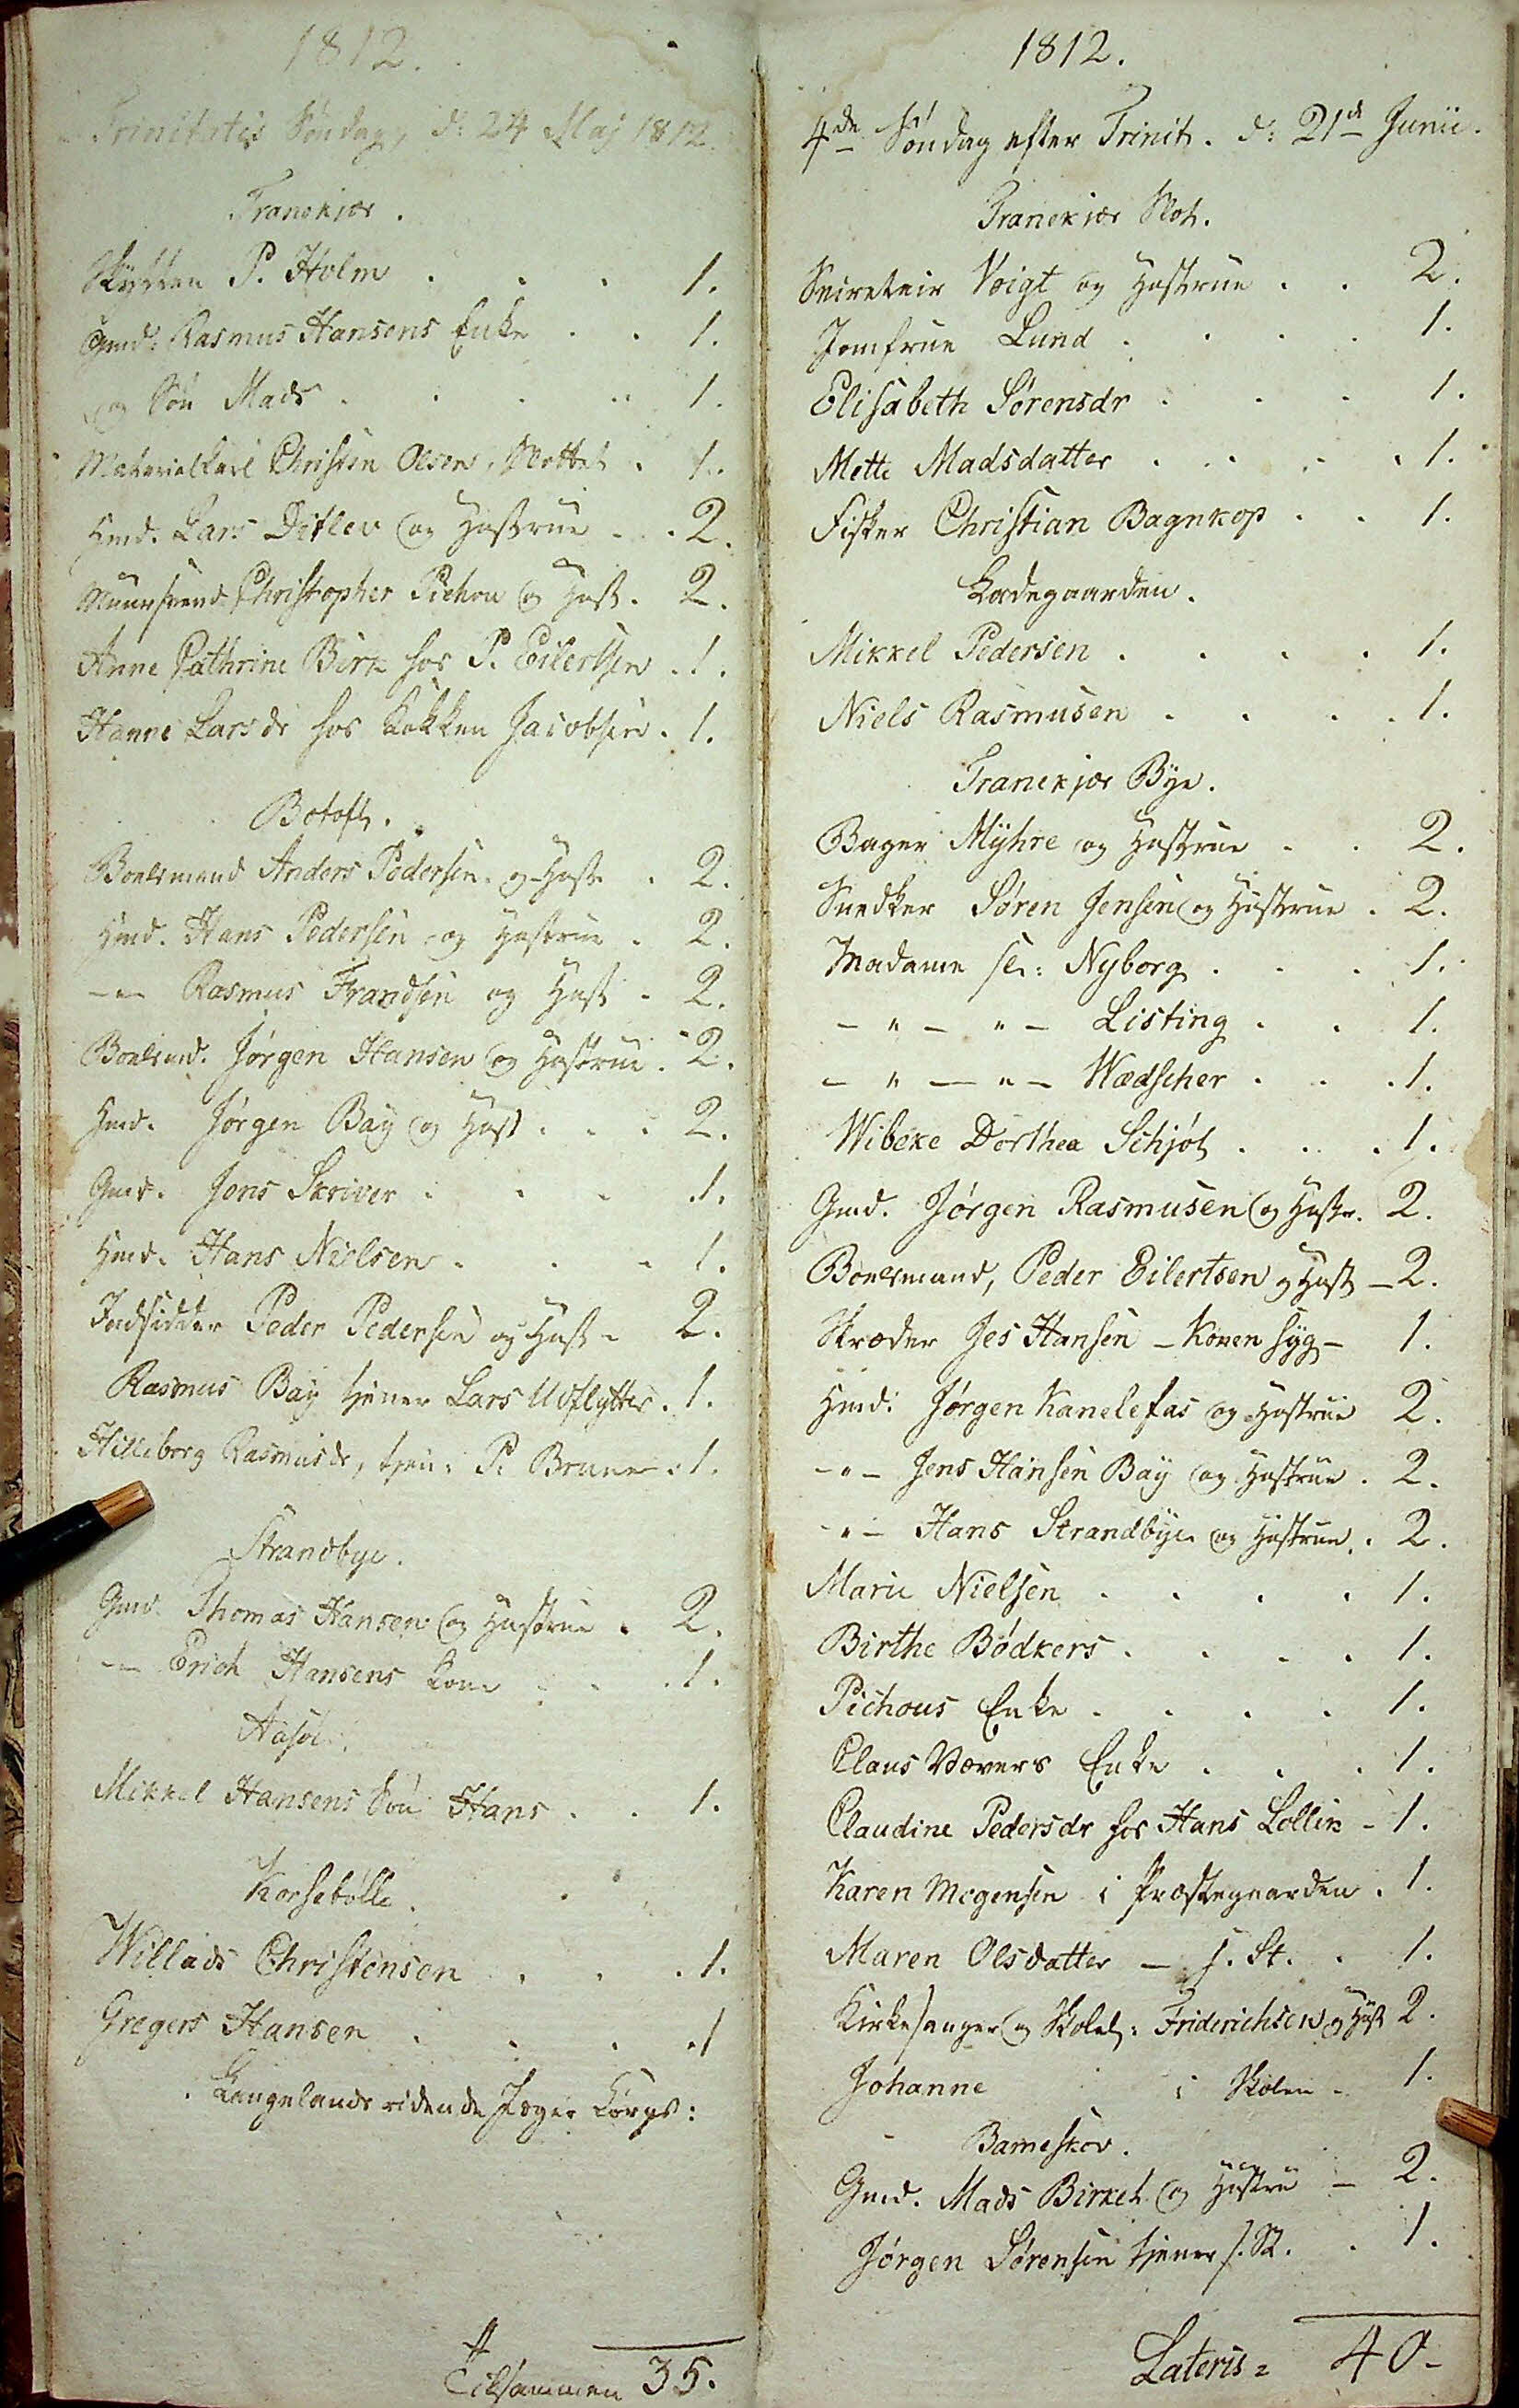1812.
Trinitatis Søndag d: 24 Maj 1812
Tranekjær
Skytten P. Holm . . . 1.
Gmd: Rasmus Hansens Enke . . 1.
og Søn Mads . . . . . 1.
Materialkarl Christen Olsen, Slottet . 1.
Hmd. Lars Ditlev og Hustrue . . . 2
Muursvend Christopher Pichou og Hust . 2.
Anne Cathrine Børk hos P. Eilertsen . 1.
Hanne Larsdr hos Kokken Jacobsen . 1.
Botoft.
Boelsmand Anders Pedersen og Hustr . 2
Hmd. Hans Pedersen og Hustrue . 2
-''- Rasmus Frandsen og Hust . 2.
Boelsmd. Jørgen Hansen og Hustrue . 2
Hmd. Jørgen Bay og Hust . . . 2.
Gmd. Jens Skriver . . . . 1.
Hmd. Hans Nielsen . . . 1.
Indsidder Peder Pedersen og Hust . 2.
Rasmus Bay tjener Lars Udflytter . 1.
Hilleborg Rasmusdr, tjen: P: Brunm . 1.
Strandbye.
Gmd. Thomas Hansen og Hustrue . 2.
-"- Erich Hansens Kone . . 1.
Aasøe
Mikkel Hansens Søn Hans . . 1.
Korsebølle
Willads Christensen . . . 1.
Gregers Hansen . . . . 1
Langelands  ridende Jæger Corps:
Tilsammen 35.
1812.
4de Søndag efter Trinit. d: 21de Junii
Tranekjær Slot.
Secreteir Voigt og Hustrue . . 2.
Jomfrue Lana . . . . 1.
Elisabeth Sørensdr . . . 1.
Mette Madsdatter . . . . . . 1.
Fisker Christian Bagnkop . . 1.
Ladegaarden.
Mikkel Pedersen . . . . 1.
Niels Rasmusen . . . . 1.
Tranekjær Bye.
Bager Myhre, og Hustrue . . 2.
Snedker Søren Jensen og Hustrue . 2.
Madame Sl: Nyborg . . . 1.
-"-"- Listing . . 1.
-"-"- Wædscher . . . 1.
Wibeke Dorthea Schjøt . . . 1.
Gmd. Jørgen Rasmusen og Hustr . 2.
Boelsmand, Peder Eilertsen og Hust - 2.
Skræder Jes Hansen - Konen syg - 1.
Hmd: Jørgen Kanelefas og Hustrue 2.
-"- Jens Hansen Bay og Hustrue . 2.
-"- Hans Strandbye og Hustrue . 2.
Marie Nielsen . . . . 1.
Birthe Bødkers . . . . 1.
Pichous Enke . . . . 1.
Claus Vævers Enke . . . 1.
Claudine Pedersdr hos Hans Lollik - 1.
Karen Mogensen i Præstegaarden . 1.
Maren Olsdatter - s. St . . 1.
Kirkesanger og Skolel: Friderichsen og Hust 2.
Johanne i Skolen - 1.
Bameskov
Gmd. Mads Birket og Hustru - 2.
Jørgen Sørensen tjener  s##. St . . 1.
Lateris = 40.
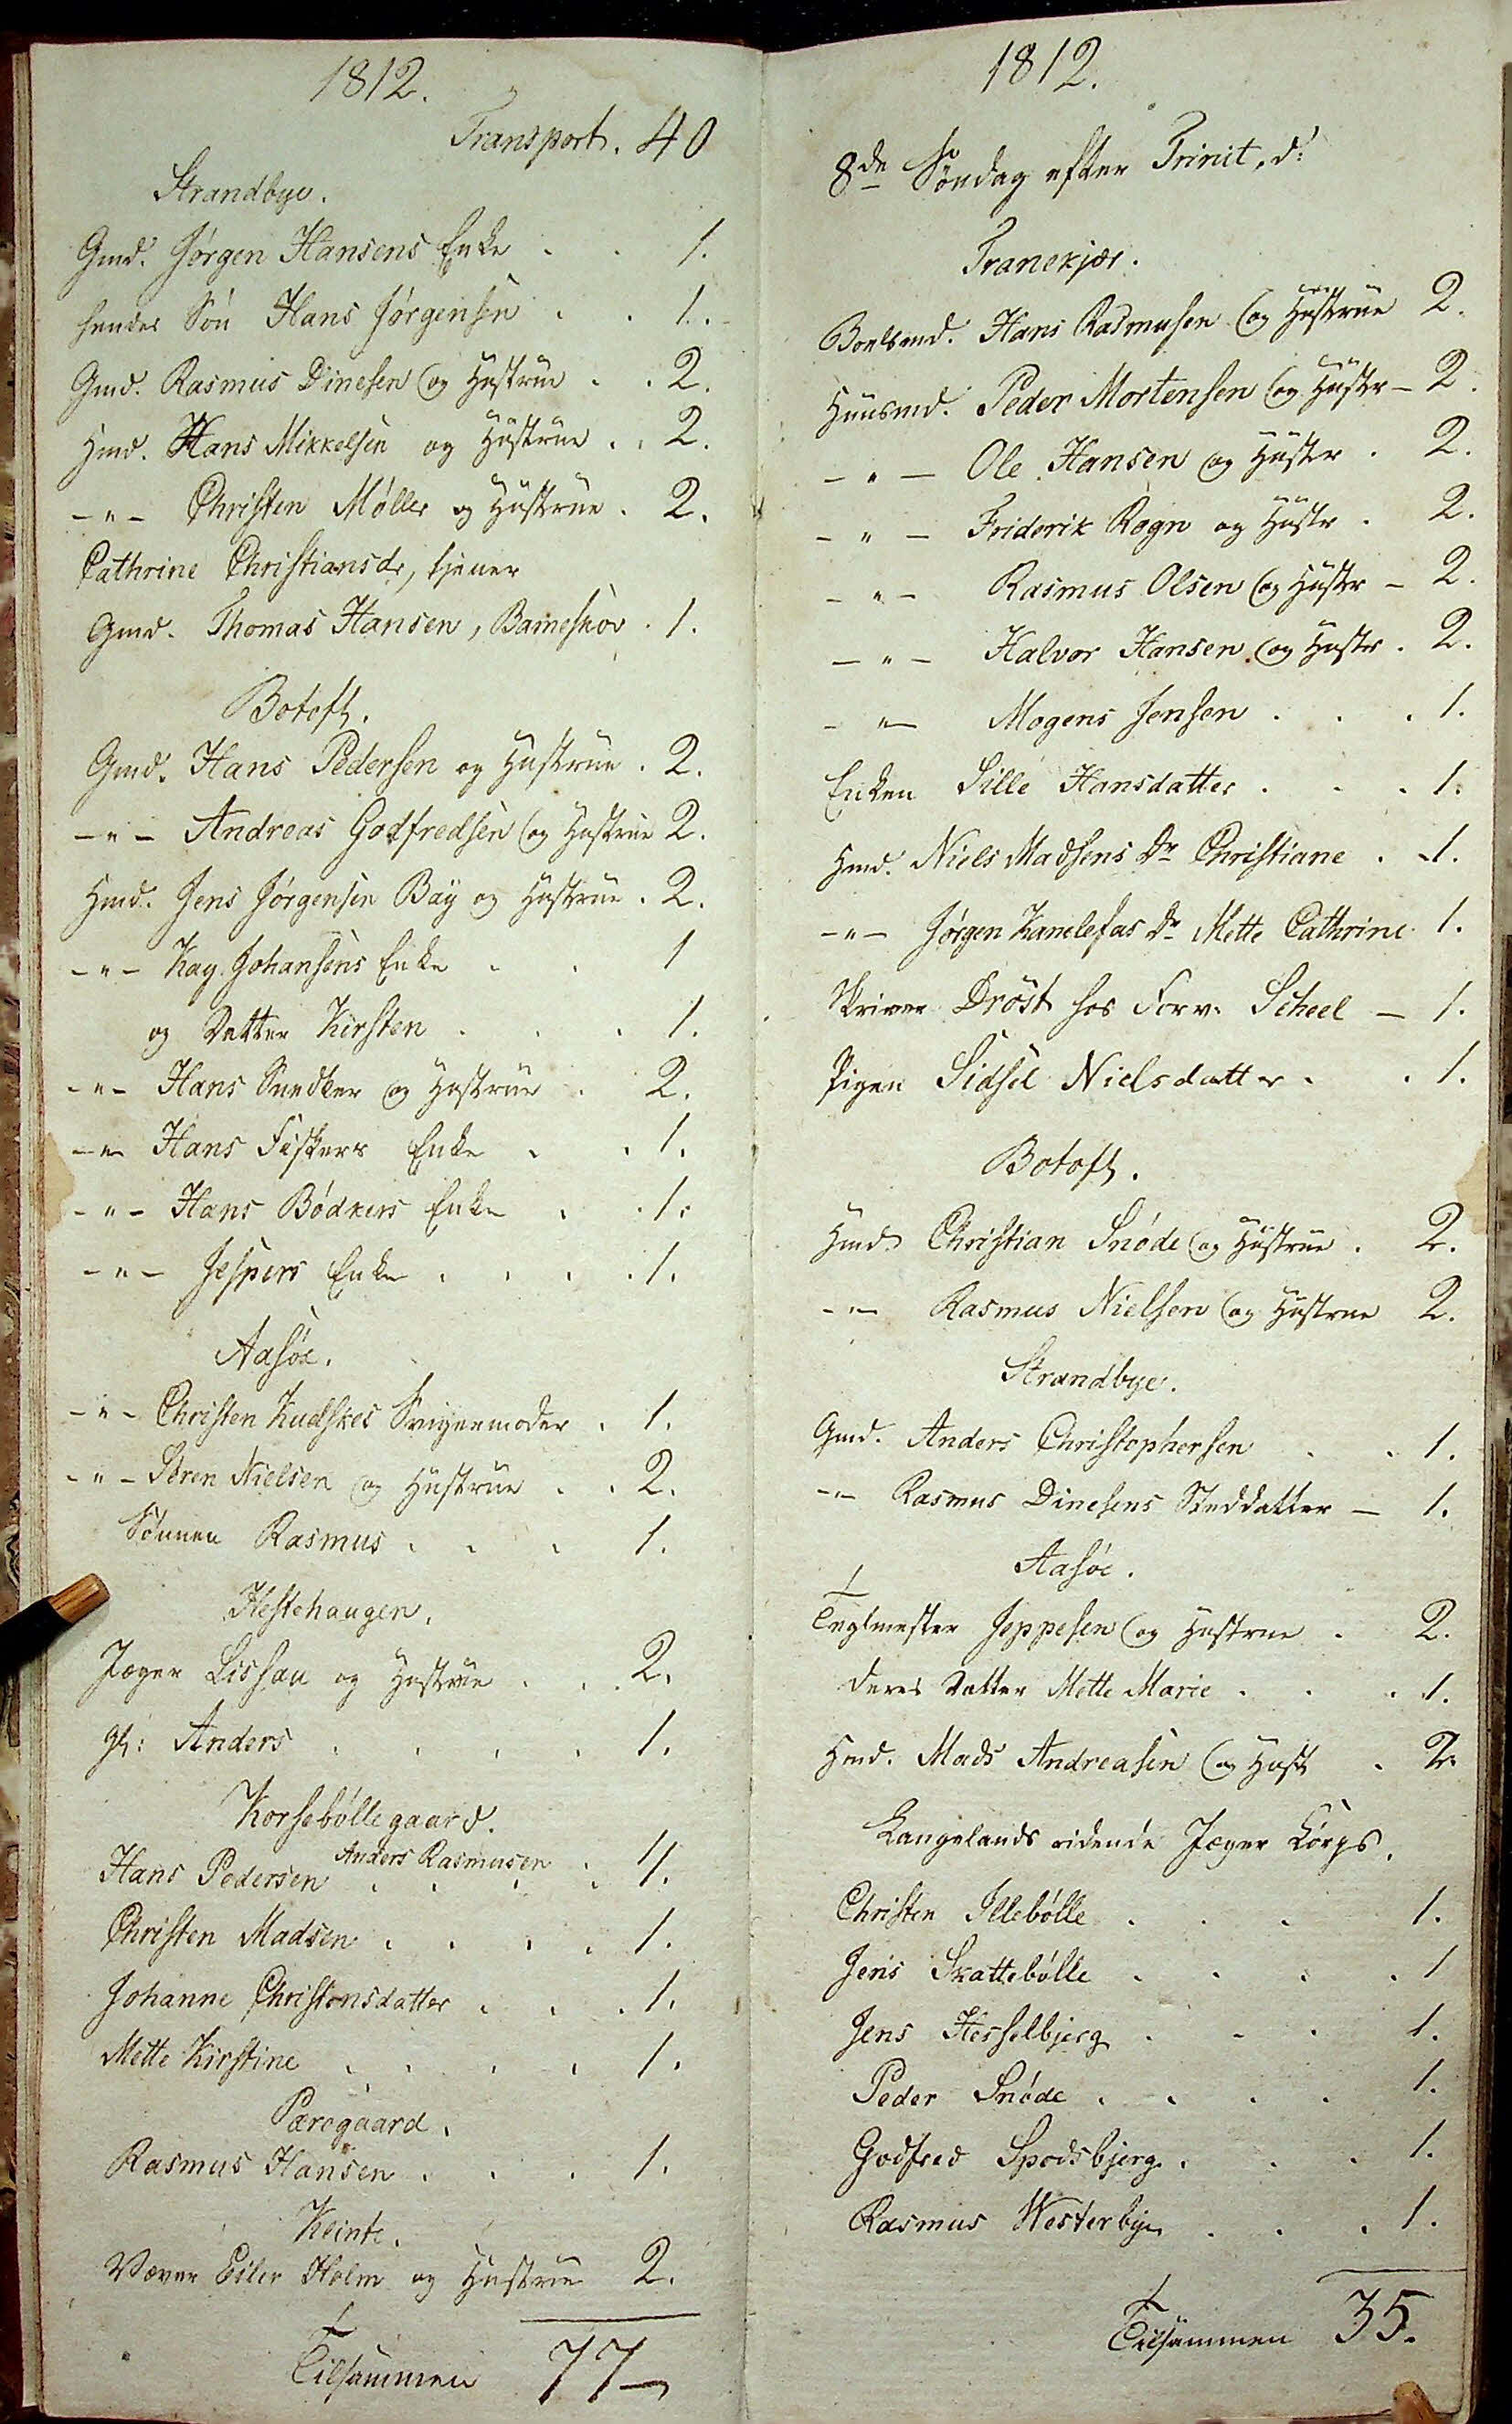1812.
Transport. 40
Strandbye.
Gmd. Jørgen Hansens Enke . . 1.
hendes Søn Hans Jørgensen . .1.
Gmd. Rasmus Dinesen og Hustrue . . 2.
Hmd. Hans Mikklsen og Hustrue . . 2.
-"- Christen Møller og Hustrue . 2.
Cathrine Christiansdr, tjener
Gmd. Thomas Hansen, Bameskov . 1.
Botoft.
Gmd. Hans Pedersen og Hustrue . 2.
-"- Andreas Godfredsen og Hustrue 2.
Hmd. Jens Jørgensen Bay og Hustrue . 2.
-"- Kay Johansens Enke . . 1
og Datter Kirsten . . . 1.
-"- Hans Snedker og Hustrue . 2
-"- Hans Fiskers Enke . . 1.
-"- Hans Bødkers Enke . . 1.
-"- Jespers Enker . . . . 1.
Aasøe.
-"- Christen Kudkes Svigermoder . 1.
-"- Søren Nielsen og Hustrue . . 2.
Sønnen Rasmus . . . 1.
Hestehaugen.
Jæger Lissau og Hustrue . . .  2.
gl: Anders . . . . 1.
Korsebøllegaard.
Anders Rasmussen . 1
Hans Pedersen . . . . 1.
Christen Madsen . . . . 1.
Johanne Christensdatter . . . 1.
Mette Kirstine . . . . 1.
Pæregaard.
Rasmus Hansen . . . 1.
Klinte.
Væver Eiler Holm og Hustrue 2
Tilsammen 77 -
1812.
8de Søndag efter Trinit, d:
Tranekjær.
Boelsmd. Hans Rasmusen og Hustrue 2.
Huusmd. Peder Mortensen og Hustru - 2
-"- Ole Hansen og Hustr. 2.
-"- Friderik Rogn og Hustr . 2.
-"- Rasmus Olsen og Hustr - 2.
-"- Halvor Hansen og Hustr . 2.
-"- Mogens Jensen . . . 1.
Enken Sille Hansdatter . . . 1
Hmd. Niels Madsens Dr Christiane . 1.
Jørgen Kanelefas Dr Mette Cathrine . 1.
Skriver Drost hos Forv: Scheel - 1.
Pigen Sidsel Nielsdatter . . 1.
Botoft
Hmd Christian Snøde og Hustrue . 2.
-"- Rasmus Nielsens og Hustrue 2.
Strandbye.
Gmd. Anders Christophersen . .1.
-"- Rasmus Dinesens Steddatter - 1.
Aasøe.
Teglmester Jeppesen og Hustrue . 2.
deres Datter Mette Marie . . . 1.
Hmd. Mads Andreasen og Hust . 2.
Langelands ridende Jæger Corps.
Christen Illebølle . . . 1.
Jens Skattebølle . . . . 1
Jens Hesselbjerg . . . 1.
Peder Snøde . . . . 1.
Godfred Spodsbjerg . . . 1.
Rasmus Westerbye . . . 1.
Tilsammen 35.